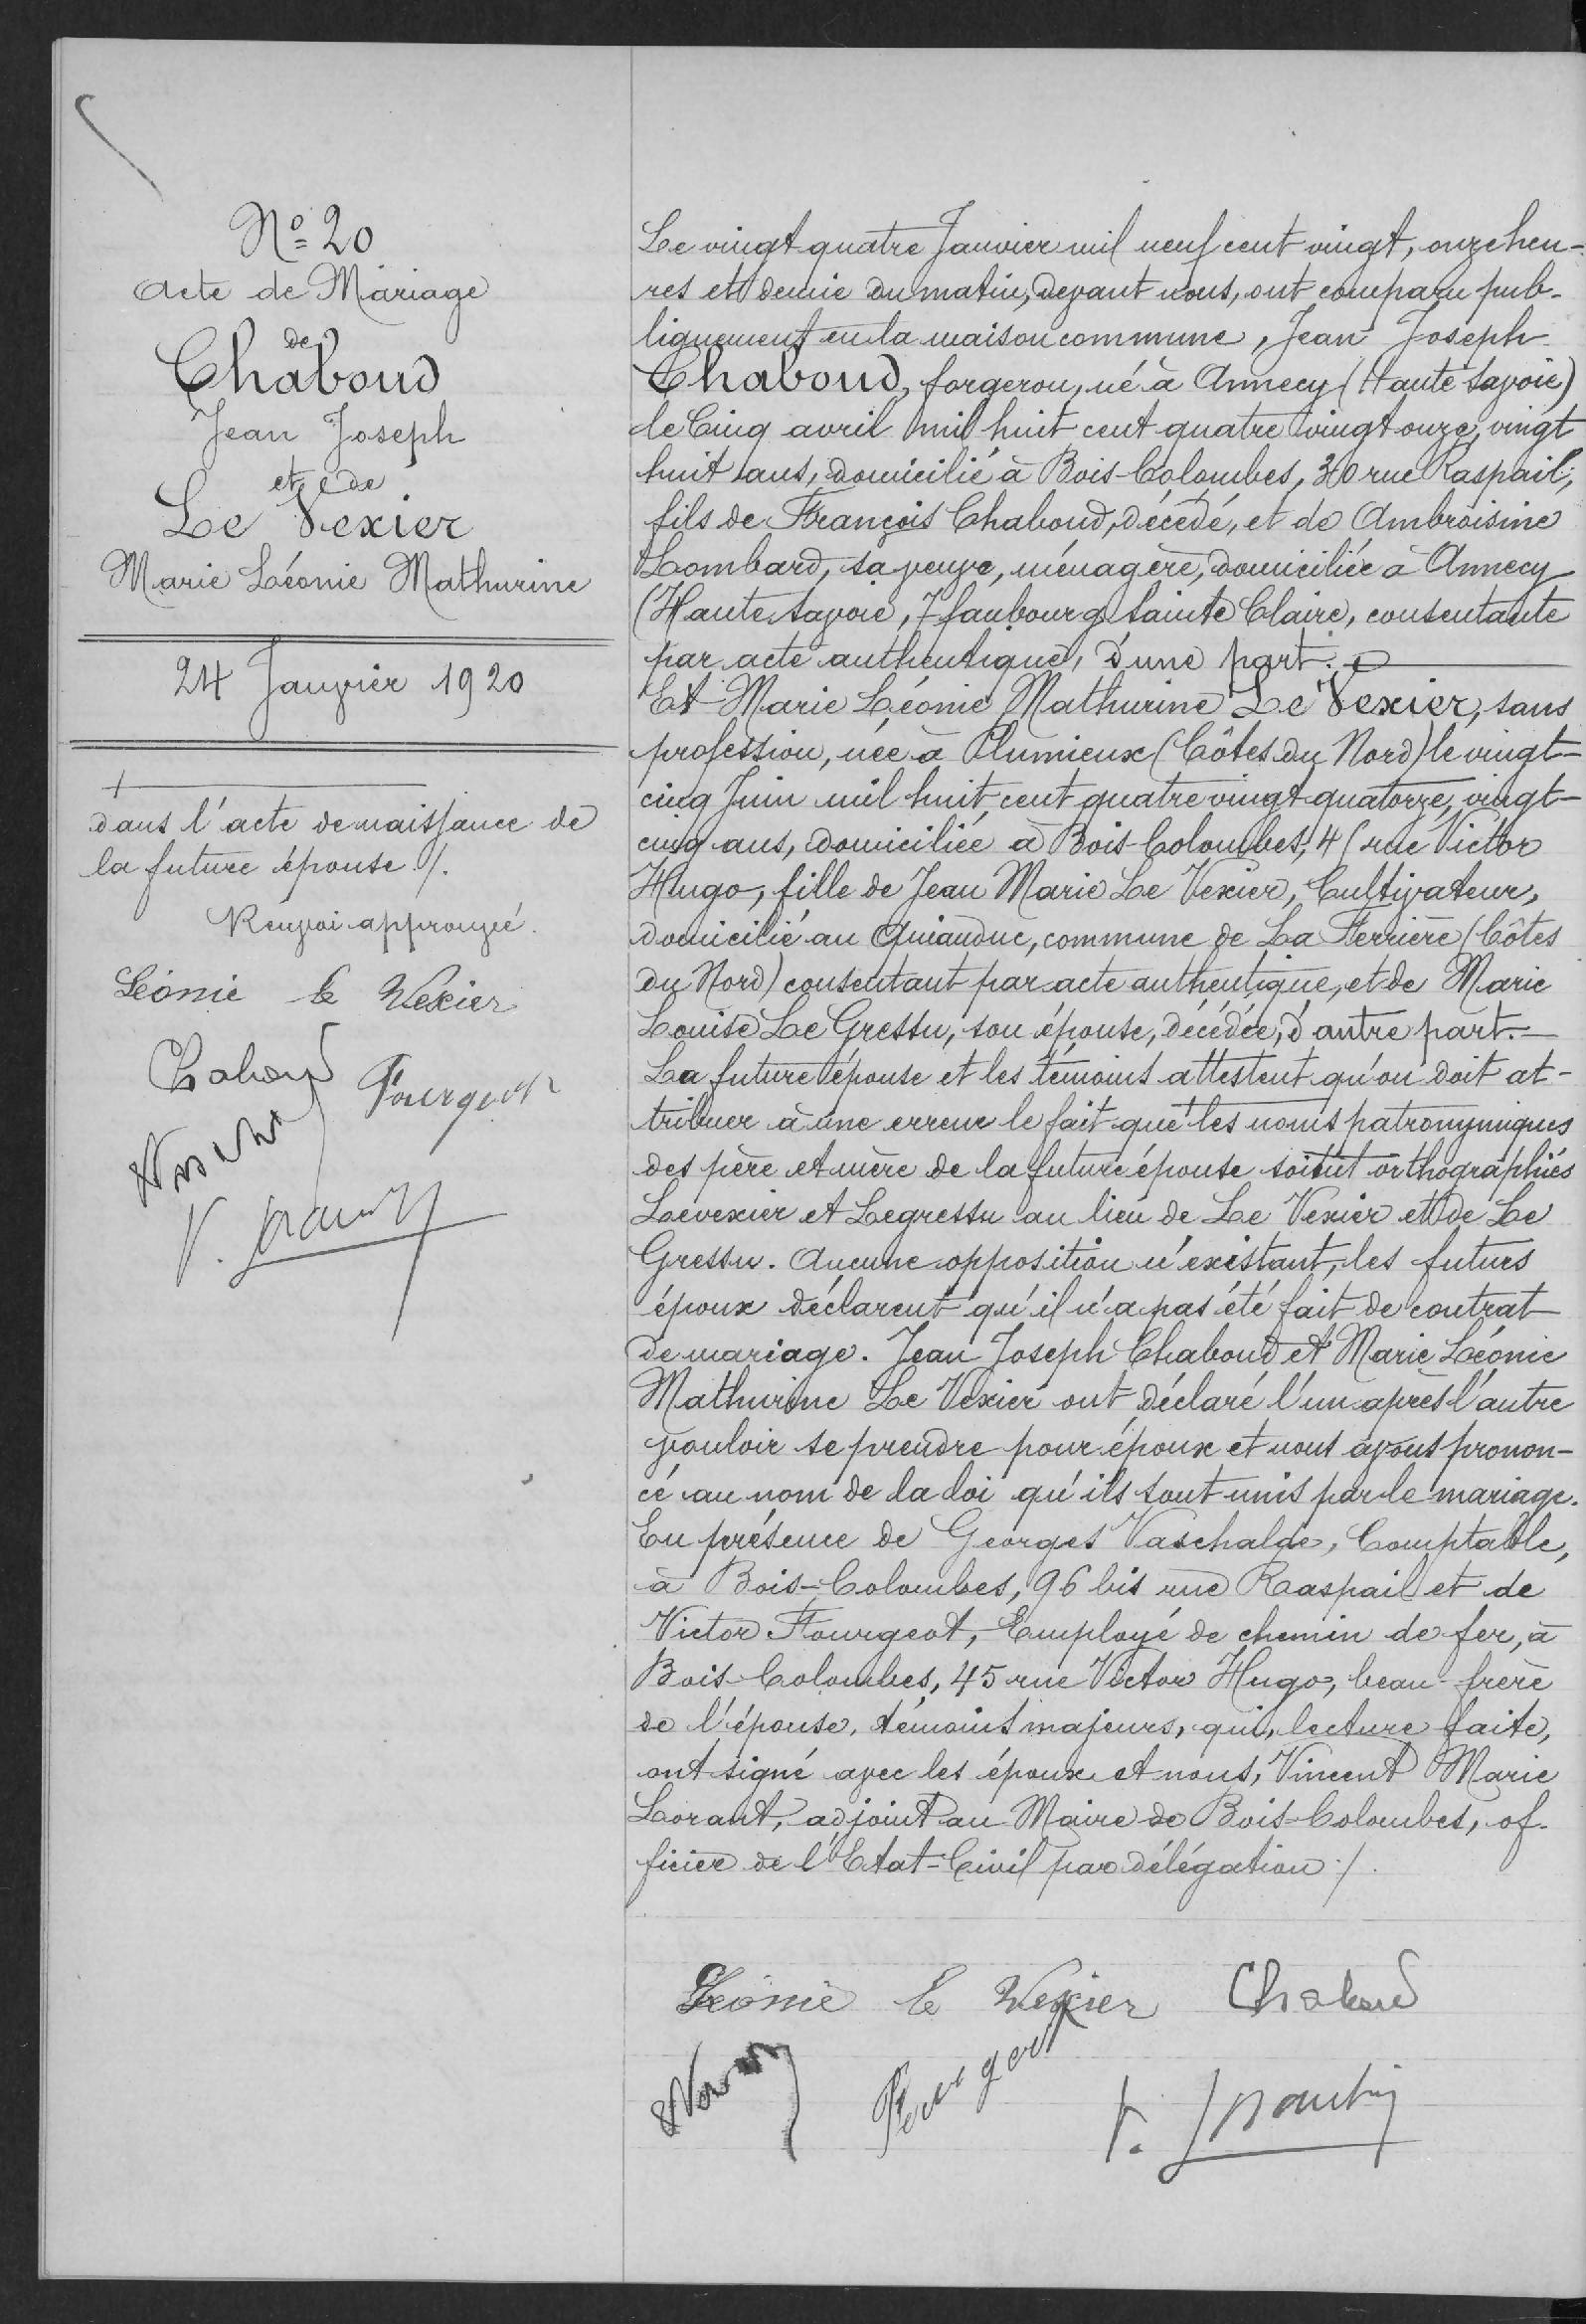N°20
acte de Mariage
de
Chaboud
Jean Joseph
et de
Le Vexier
Marie Léonie Mathurine
Le vingt quatre Janvier mil neuf cent vingt, onze heu-
res et demie du matin, devant nous, ont comparu pub-
liquement en la maison commune, Jean Joseph
Charbond, forgeron, né à Annecy (Haute Savoie)
le Cinq avril mil huit cent quatre vingt onze, vingt
huit ans, domicilié à Bois-Colombes, 360 rue Raspail,
fils de François Chaboud, décédé, et de Ambroisine
Lombard, sa veuve, ménagère, domiciliée à Annecy
(Haute Savoie), 7 faubourg Sainte Claire, consentante
par acte authentique, d'une part.-
Et Marie Léonie Mathurine Le Vexier, sans
profession, née à Plumieux (Côtes du Nord) le vingt
cinq Juin mil huit cent quatre vingt quatorze, vingt-
cinq ans, domicilie à Bois Colombe, 4 rue Victor
Hugo, fille de Jean Marie Le Vexier, Cultivateur,
domicilié au Quiaudue, commune de la Ferrière (Côtes
du Nord) consentant par acte authentique, et de Marie
Louise Le Gressic, son épouse, décédée, d'autre part.-
La future épouse et les témoins attestent qu'on doit at-
tribuer à une erreur le fait que les noms patronymiques
des père et mère de la future épouse soient orthographiés
Laevexier et Legressu au lieu de Le Vexier et de Le
Gressu. Aucune opposition n'existant, les futurs
époux déclarent qu'il n'a pas été fait de contrat
de mariage. Jean Joseph Chaboud et Marie Léonie
Mathurine Le Vexier ont déclaré l'un après l'autre
vouloir se prendre pour époux et nous avons pronon-
cé au nom de la loi qu'ils sont unis par le mariage.
En présence de Georges Vasehalde, comptable,
à Bois Colombes, 96 bis rue Raspail et de
Victor Fourgeot, Employé de chemin de fer, à
Bois Colombes, 45 rue Victor Hugo, beau frère
de l'épouse, témoins majeurs, qui, lecture faite,
ont signé avec les époux et nous, Vincent Marie
Loraut, adjoint au Maire de Bois Colombes, of
ficier de l'Etat Civil par délégation
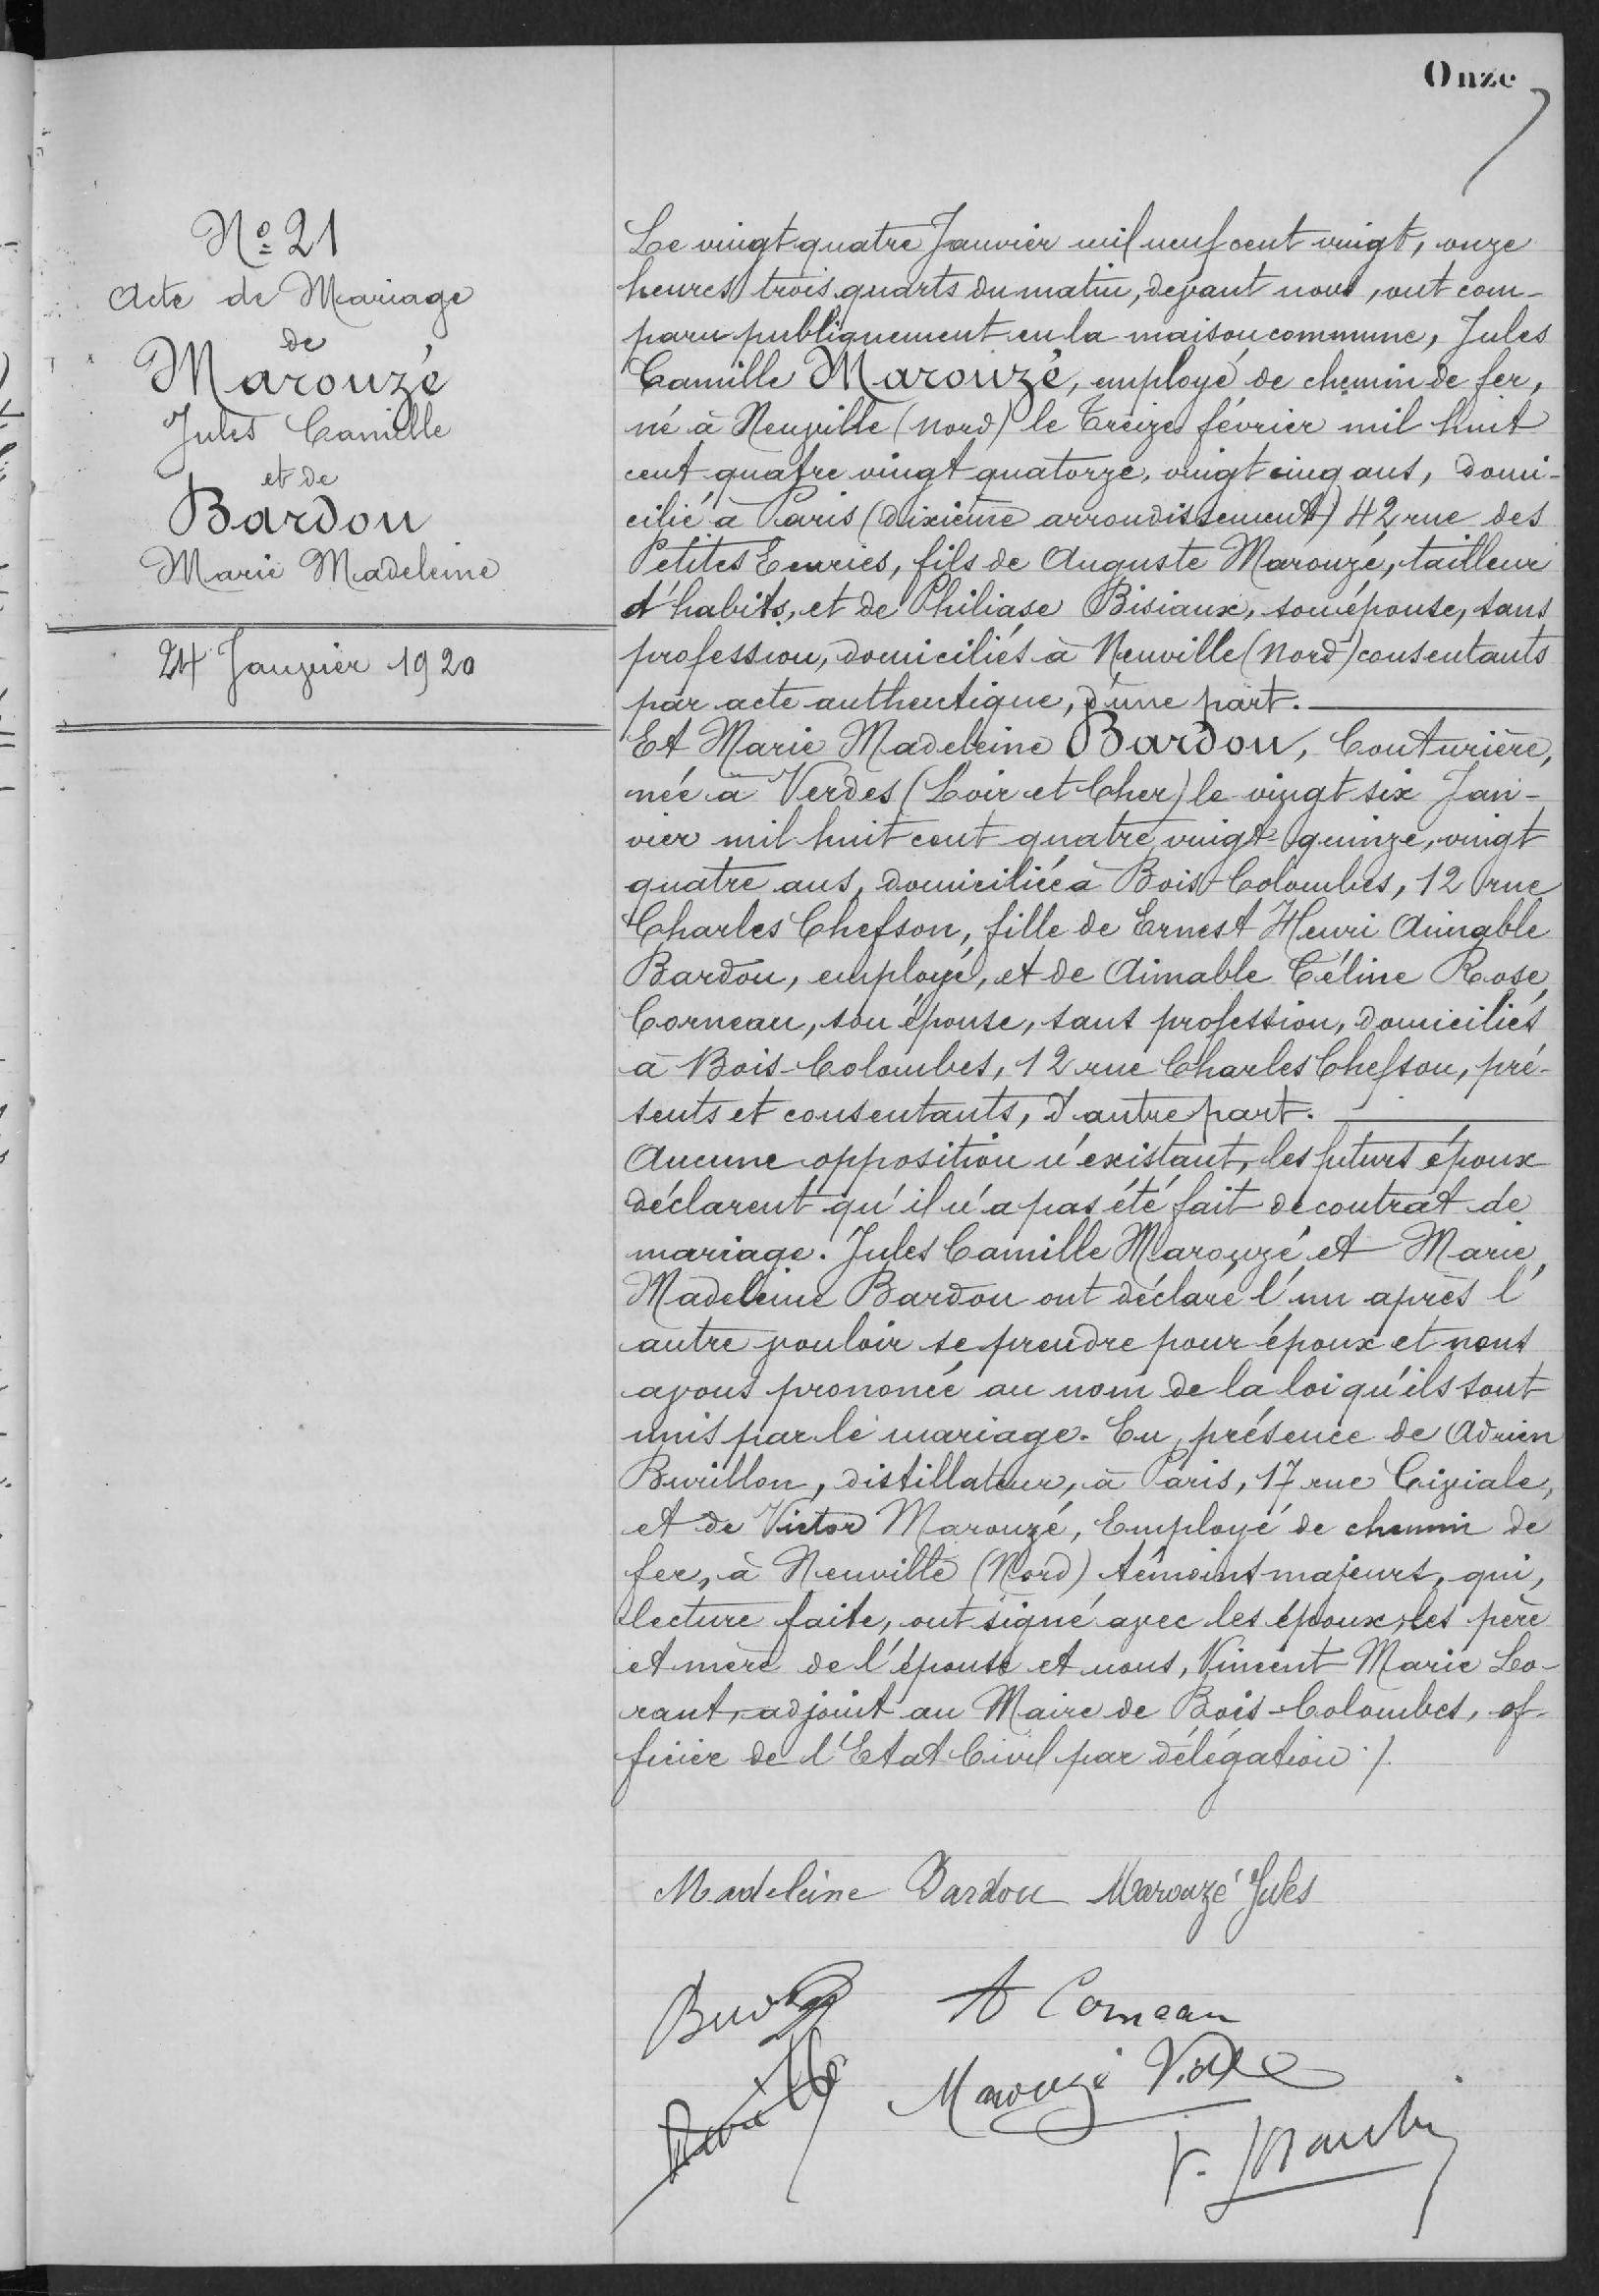N°21
Acte de Mariage
de
Marouzé
Jules Camille
et de
Bardou
Marie Madeleine
24 Janvier 1920
Le vingt quatre janvier mil neuf cent vingt, onze
heures trois quarts du matin, devant nous, ont com-
paru publiquement en la maison commune, Jules
Camille Marouzé, employé de chemin de fer,
né à Neuville (Nord) le treize février mil huit
cent quatre vingt quatorze, vingt-cinq ans, domi-
cilié à Paris (dixième arrondissement) 42, rue des
Petites Curies, fils de Auguste Marouzé, tailleur
d'habits et de Philiase Bisiaux, son épouse, sans
profession, domiciliés à Neuville (Nord) consentants
par acte authentique, d'une part.-
Et Marie Madeleine Bardou, couturière,
née à Verdes (Loir et Cher) le vingt six Jan-
vier mil huit cent quatre vingt quinze, vingt
quatre ans, domiciliée à Bois Colombes, 12 rue
Charles Chefson, fille de Ernest Henri Aimable
Bardou, employé, et de Aimable Céline Rose
Corneau, son épouse, sans profession, domiciliés
à Bois-Colombes, 12 rue Charles Chefson, pré-
sents et consentants, d'autre part.-
Aucune opposition n'existant, les futurs époux
déclarent qu'il n'a pas été fait de contrat de
mariage. Jules Camille Marouz et Marie
Madeleine Bardou ont déclaré l'un après l'
autre vouloir se prendre pour époux et nous
avons prononcé au nom de la loi qu'ils sont
unis par le mariage. En présence de Adrien
Burillon, distillateur, à Paris, 17 rue Criviale,
et de Victor Marouzé, employé de chemin de
fer, à Neuvillé (Nord) témoins majeurs qui,
lecture faite, ont signé avec les époux, les père
et mère de l'épouse et nous, Vincent Marie Lo-
rant, adjoint au Maire de Bois-Colombes, of-
ficier de l'Etat Civil par délégation ./.